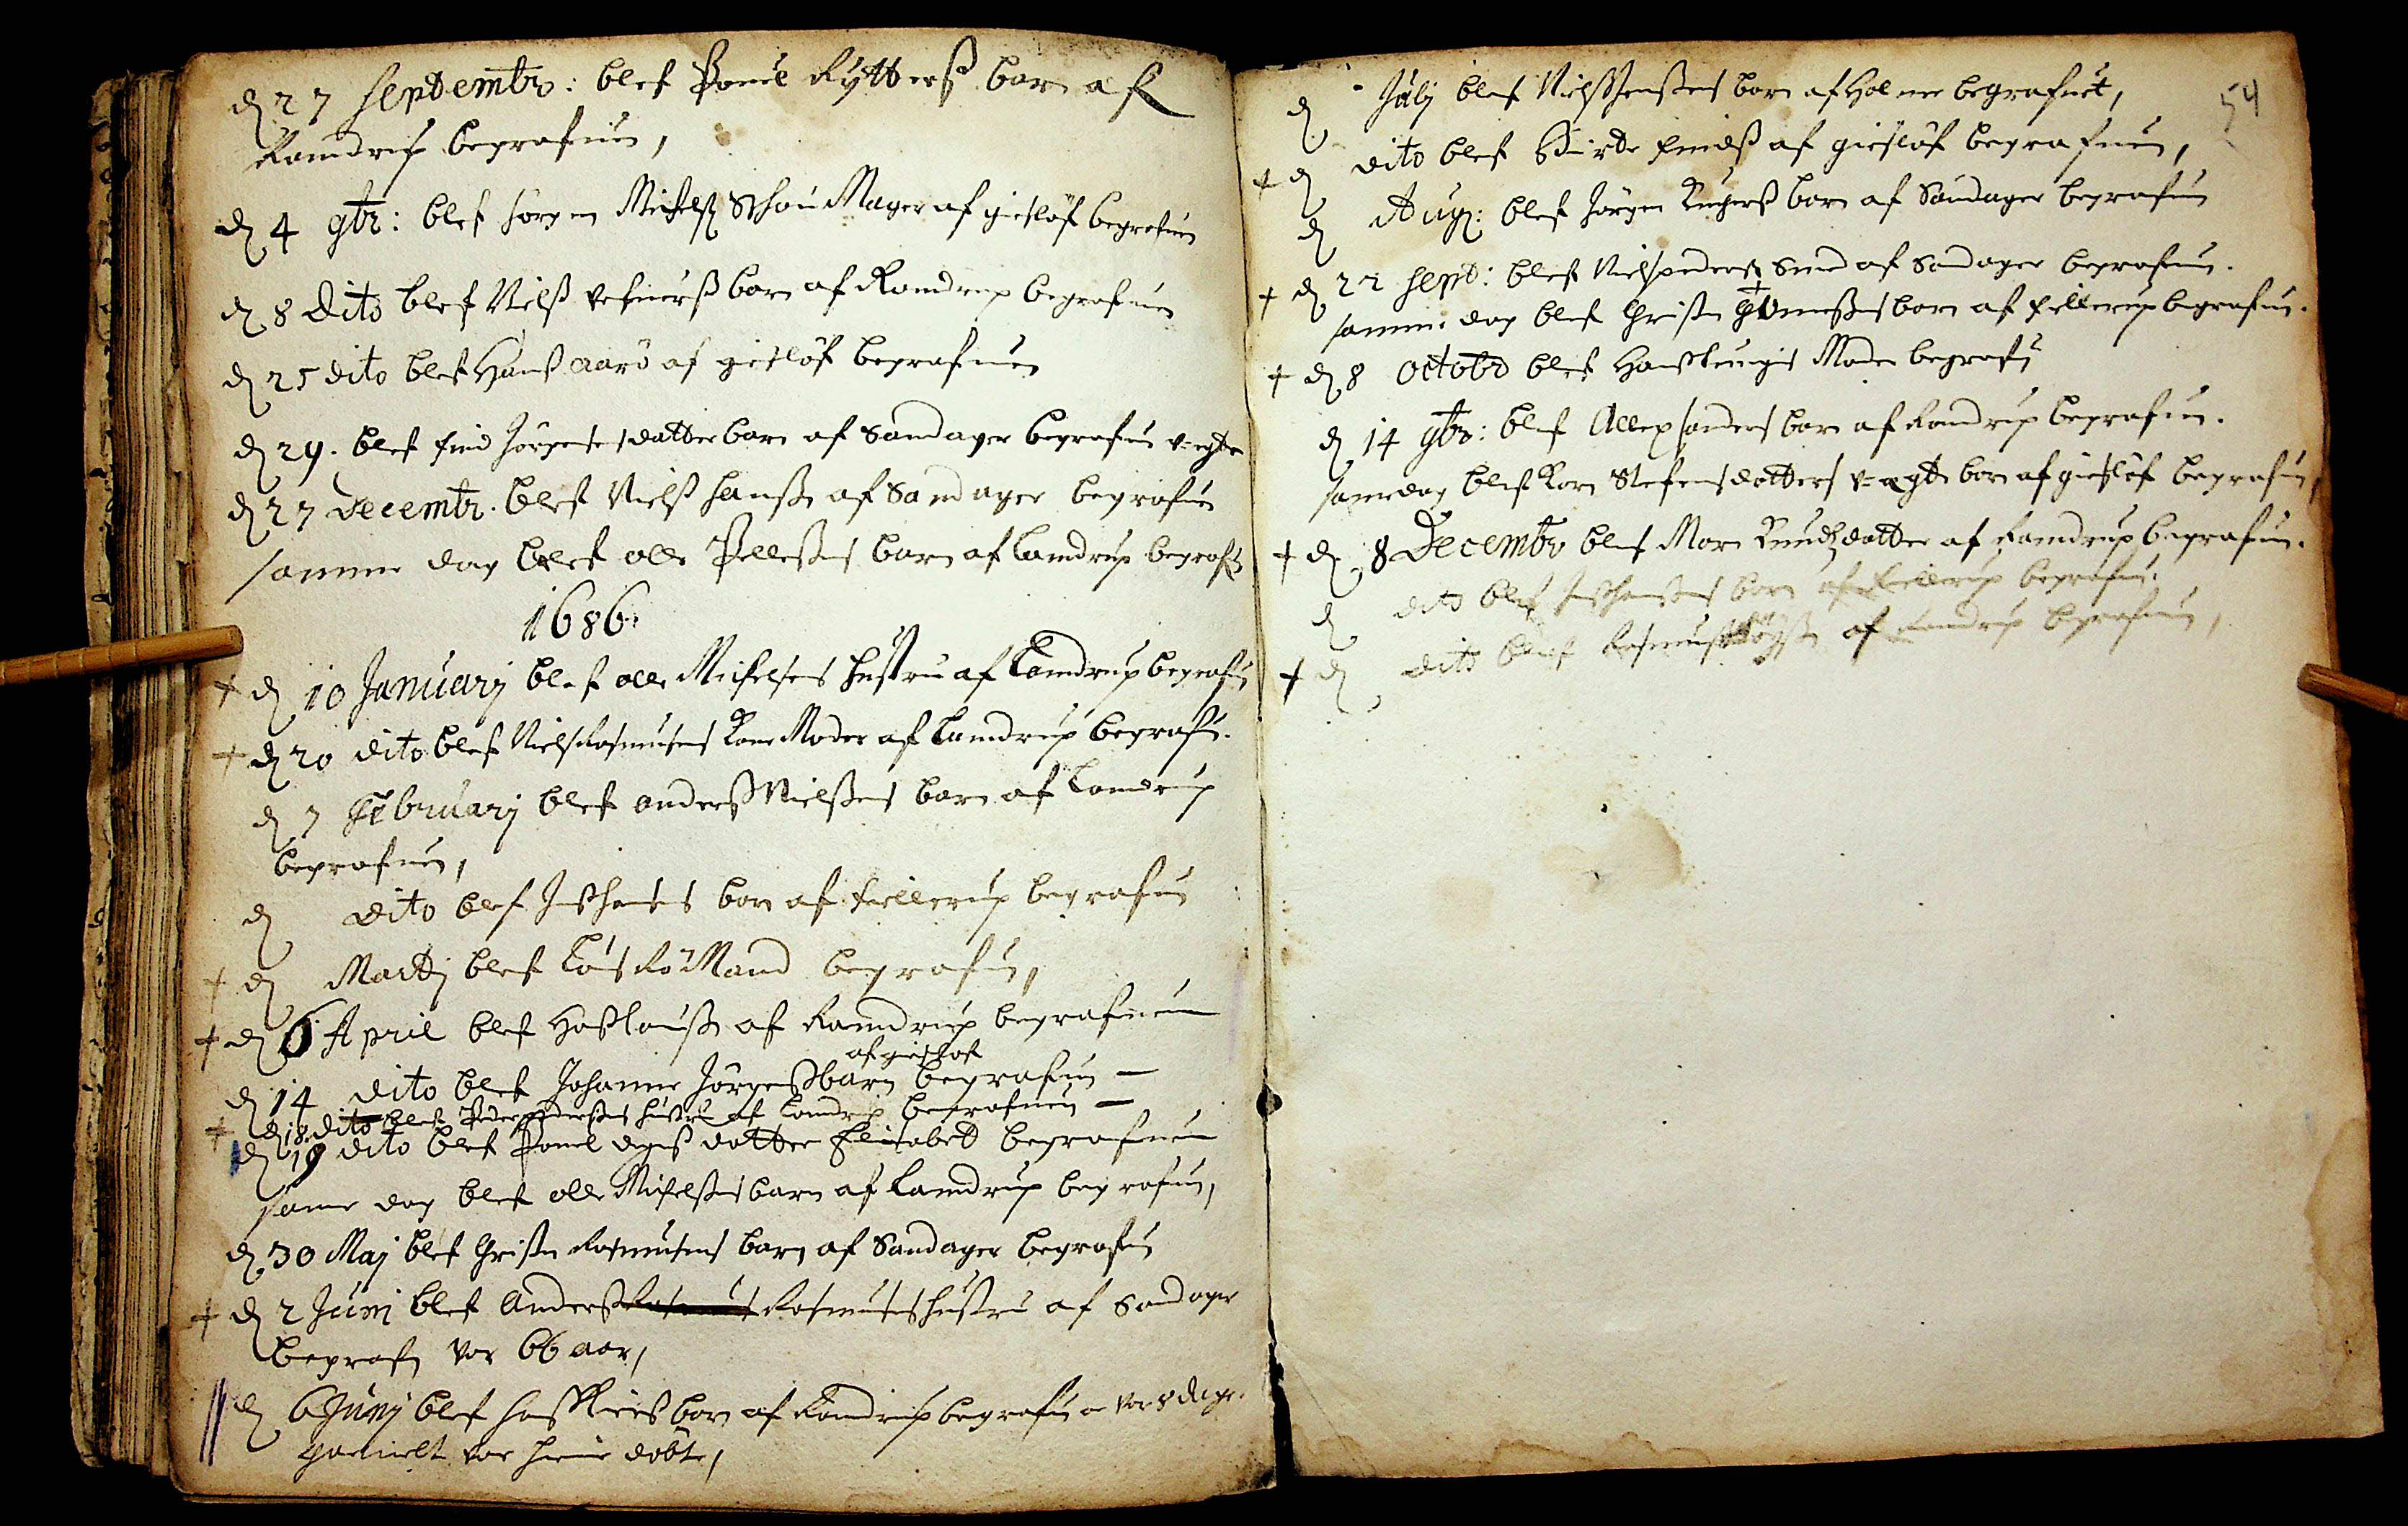d 27 Septembr: blef Pouel Rytterss barn af
Ramdrup begrafuen,
d 4 9br: blef Jørgen Michels Shou Mager af Girsløf begrfuen
d 8 Dito blef Niels Vefuerss barn af Ramdrup begrafuen
d 25 dito blef Hanss Aars af giesløf begrafuen
d 29. blef find Jørgensens datter barn aff Sandager begrafuen v=egte
d 27 Decembr. blef Nielss Hansen af Søndager begrafuen
samme dag blef Olle Pellessens barn af Lamdrup begraf##
1686.
d 10 Januarij blef olle Michelsens Hustrue af Lamdrup begrafuen
d 20 dito blef Niels Rasmusens Kone Moder af Lamdrup begraf.
d 7 Februarij blef Anderss Nielssens barn af Lamderup
begrafuen,
d dito blef Jens Hansens born aff Fiellerup begrafuen
d Martij blef Laus Rø Mand begrafuen,
d 6 April bleff Hans laussen af Ramdrup begrafuen
af gieslof
d 14 dito blef Johanne Jørgenss barn begrafuen
d 18 dito blef Peder pedersens hustru af Lamdrup begrafuen
d 19 dito blef Pouel degns datter Elisabet begraffuen
samme dag blef olle Michelssens barn af Lamdrup begrafuen
d 30 Maj blef Christen Rasmusens barn af Sandager begrafuen
d 2 Juni blef Anderss Rasmus Rasmusens hustru af Sandager
begraf var 66 Aar,
d 6 Junij blef hans Nielss barn af Ramdrup begraffuet oc var 8 dage
gammelt var hiem døbte,
54
d Julj blef Nielss Jenssens barn af Holme begrafuet
d dito bleff Birte Finds af giesløf begrafuen,
d Aug: bleff Jørgen Kucherss barn af Sadager begraffuen
d. 22 Sept: blef Niels pedersens Smed af Sandager begrafuen
samme dag blef Christen chlemessens barn af Fiellerup bgrufuen
d 8 Octobr blef Hans ##ugis Moder begraf##
d. 14 9br: blef Allex sanders barn af Ramdrup begrafuen.
sammedag blef Karn Stefensdatters v=ægte barn af giesløf begrafuen
d 8 Decembr blef Marn Knudtzdatter af Ramdrup begrafuen
d dito blef Jens Hanssens barn af Fiellerup begrafuen
d dito blef Rasmus tøyssens af Ramdrup begrafuen